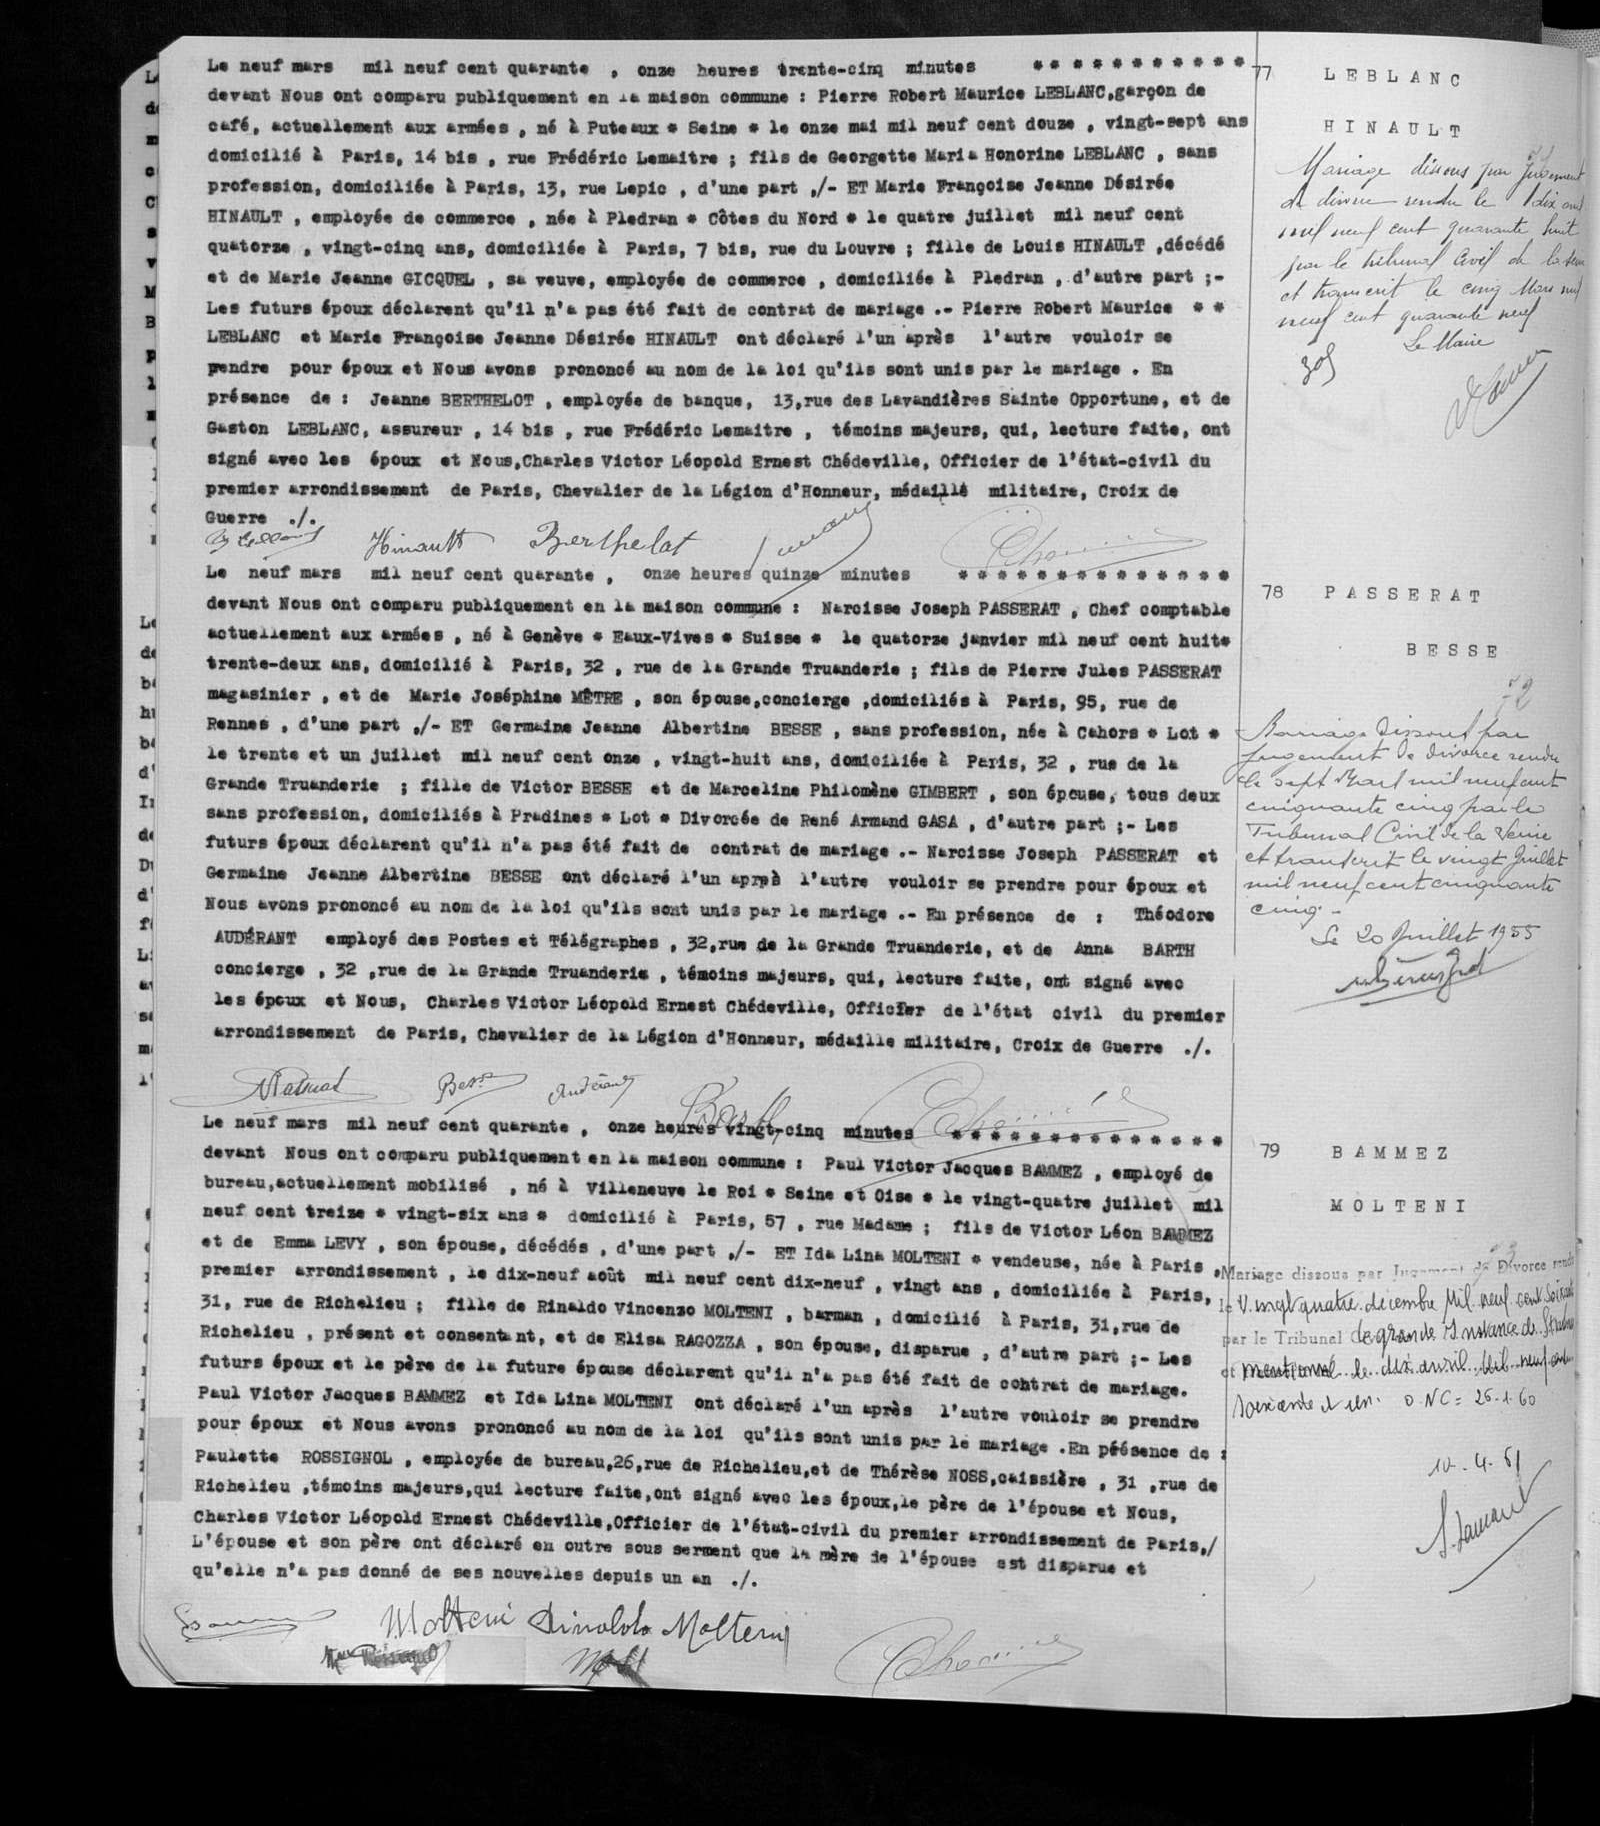Le neuf mars mil neuf cent quarante, onze heures trente-cinq minutes ****
devant Nous ont comparu publiquement en la maison commune: Pierre Robert Maurice LEBLANC, garçon de
café, actuellement aux armés, né à Puteux * Seine * le onze mai mil neuf cent douze, vingt-sept ans
domicilié à Paris, 14 bis, rue Frédéric Lemaitre; fils de Georgette Maria Honorine LEBLANC, sans
profession, domiciliée à Paris, 13, rue Lepic, d'une part ,/-ET Marie Françoise Jeanne Désirée
HINAULT, employée de commerce, née à Pledran * Côtes du Nord * le quatre juillet mil neuf cent
quatorze, vingt-cinq ans, domiciliée à Paris, 7 bis, rue du Louvre; fille de Louis HINAULT, décédé
et de Marie Jeanne GICQUEL, sa veuve, employée de commerce, domiciliée à Pledran, d'autre part;
Les futurs époux déclarent qu'il n'a pas été fait de contrat de mariage Pierre Robert Maurice **
LEBLANC et Marie Françoise Jeanne Désirée HINAULT ont déclaré l'un après l'autre vouloir se
prendre pour époux et Nous avons prononcé au nom de la loi qu'ils sont unis par le mariage. En
présence de: Jeanne BERTHELOT, employée de banque, 13, rue des Lavandières Sainte Opportune, et de
Gaston LEBLANC, assureur, 14 bis, rue Frédéric Lemaitre, témoins majeurs, qui, lecture faite, ont
signé avec les époux et Nous, Charles Victor Léopold Ernest Chédeville, Officier de l'état-civil du
premier arrondissement de Paris, Chevalier de la Légion d'Honneur, médaillé militaire, Croix de
Guerre ./.
Le neuf mars mil neuf cent quarante, onze heures quinze minutes ****
devant Nous ont comparu publiquement en la maison commune: Narcisse Joseph PASSERAT, Chef comptable
actuellement aux armées, né à Genève * Eaux-Vives * Suisse * le quatorze janvier mil neuf cent huit *
trente-deux ans, domicilié à Paris, 32, rue de la Grande Truanderie; fils de Pierre Jules PASSERAT
magasinier, et de Marie Joséphine METRE, son épouse, concierge, domiciliés à Paris, 95, rue de
Rennes, d'une part ,/-ET Germaine Jeanne Albertine BESSE, sans profession, née à Cahors * Lot *
le trente et un juillet mil neuf cent onze, vingt-huit ans, domiciliée à Paris, 32, rue de la
Grande-Truanderie; fille de Victor BESSE et de Marceline Philomène GIMBERT, son épouse, tous deux
sans profession, domiciliés à Pradines * Lot * Divorcéede rené Armand GASA, d'autre part ;-Les
futurs époux déclarent qu'il n'a pas été fait de contrat de mariage .-Narcisse Joseph PASSERAT et
Germaine Jeanne Albertine BESSE ont déclaré l'un après l'autre vouloir se prendre pour époux et
Nous avons prononcé au nom de la loi qu'ils sont unis par le mariage .-En présence de: Théodore
AUDERANT employé des Postes et Télégraphes, 32, rue de la Grande Truanderie, et de Anna BARTH
concierge, 32, rue de la Grande Truanderie, témoins majeurs, qui, lecture faite, ont signé avec
les époux et Nous, Charles Victor Léopold Ernest Chédeville, Officier de l'état civil du premier
arrondissement de Paris, Chevalier de la Légion d'Honneur, médaille militaire, Croix de Guerre ./.
Le neuf mars mil neuf cent quarante, onze heures vingt-cinq minutes ****
devant Nous ont comparu publiquement en la maison commune: Paul Victor Jacques BAMMEZ, employé de
bureau, actuellement mobilisé, né à Villeneuve le Roi * Seine et Oise * le vingt-quatre juillet mil
neuf cent treize * vingt-six ans * domicilié à Paris, 57, rue Madame; fils de Victor Léon BAMMEZ
et de Emma LEVY, son épouse, décédés, d'une part ,/-ET Ida Lina MOLTENI * vendeuse, née à Paris,
premier arrondissement, le dix-neuf août mil neuf cent dix-neuf, vingt ans, domiciliée à Paris,
31, rue de Richelieu; fille de Rinaldo Vincenzo MOLTENI, barman, domicilié à Paris, 31, rue de
Richelieu, présent et consentant, et de Elisa RAGOZZA, son épouse, disparue, d'autre part ;-Les
futurs époux et le père de la future épouse déclarent qu'il n'a pas été fait de contrat de mariage.
Paul Victor Jacques BAMMEZ et Ida Lina MOLTENI ont déclaré l'un après l'autre vouloir se prendre
pour époux et Nous avons prononcé au nom de la loi qu'ils sont unis par le mariage. En présence de:
Paulette ROSSIGNOL, employée de bureau, 26, rue de Richelieu, et de Thérèse NOSS, caissière, 31, rue de
Richelieu, témoins majeurs, qui lecture faite, ont signé avec les époux, le père de l'épouse et Nous,
Charles Victor Léopold Ernest Chédeville, Officier de l'état-civildi premier arrondissement de Paris ,/
L'épouse et son père ont déclaré en outre sous serment que la mère de l'épouse est disparue et
qu'elle n'a pas donné de ses nouvelles depuis un an ./.
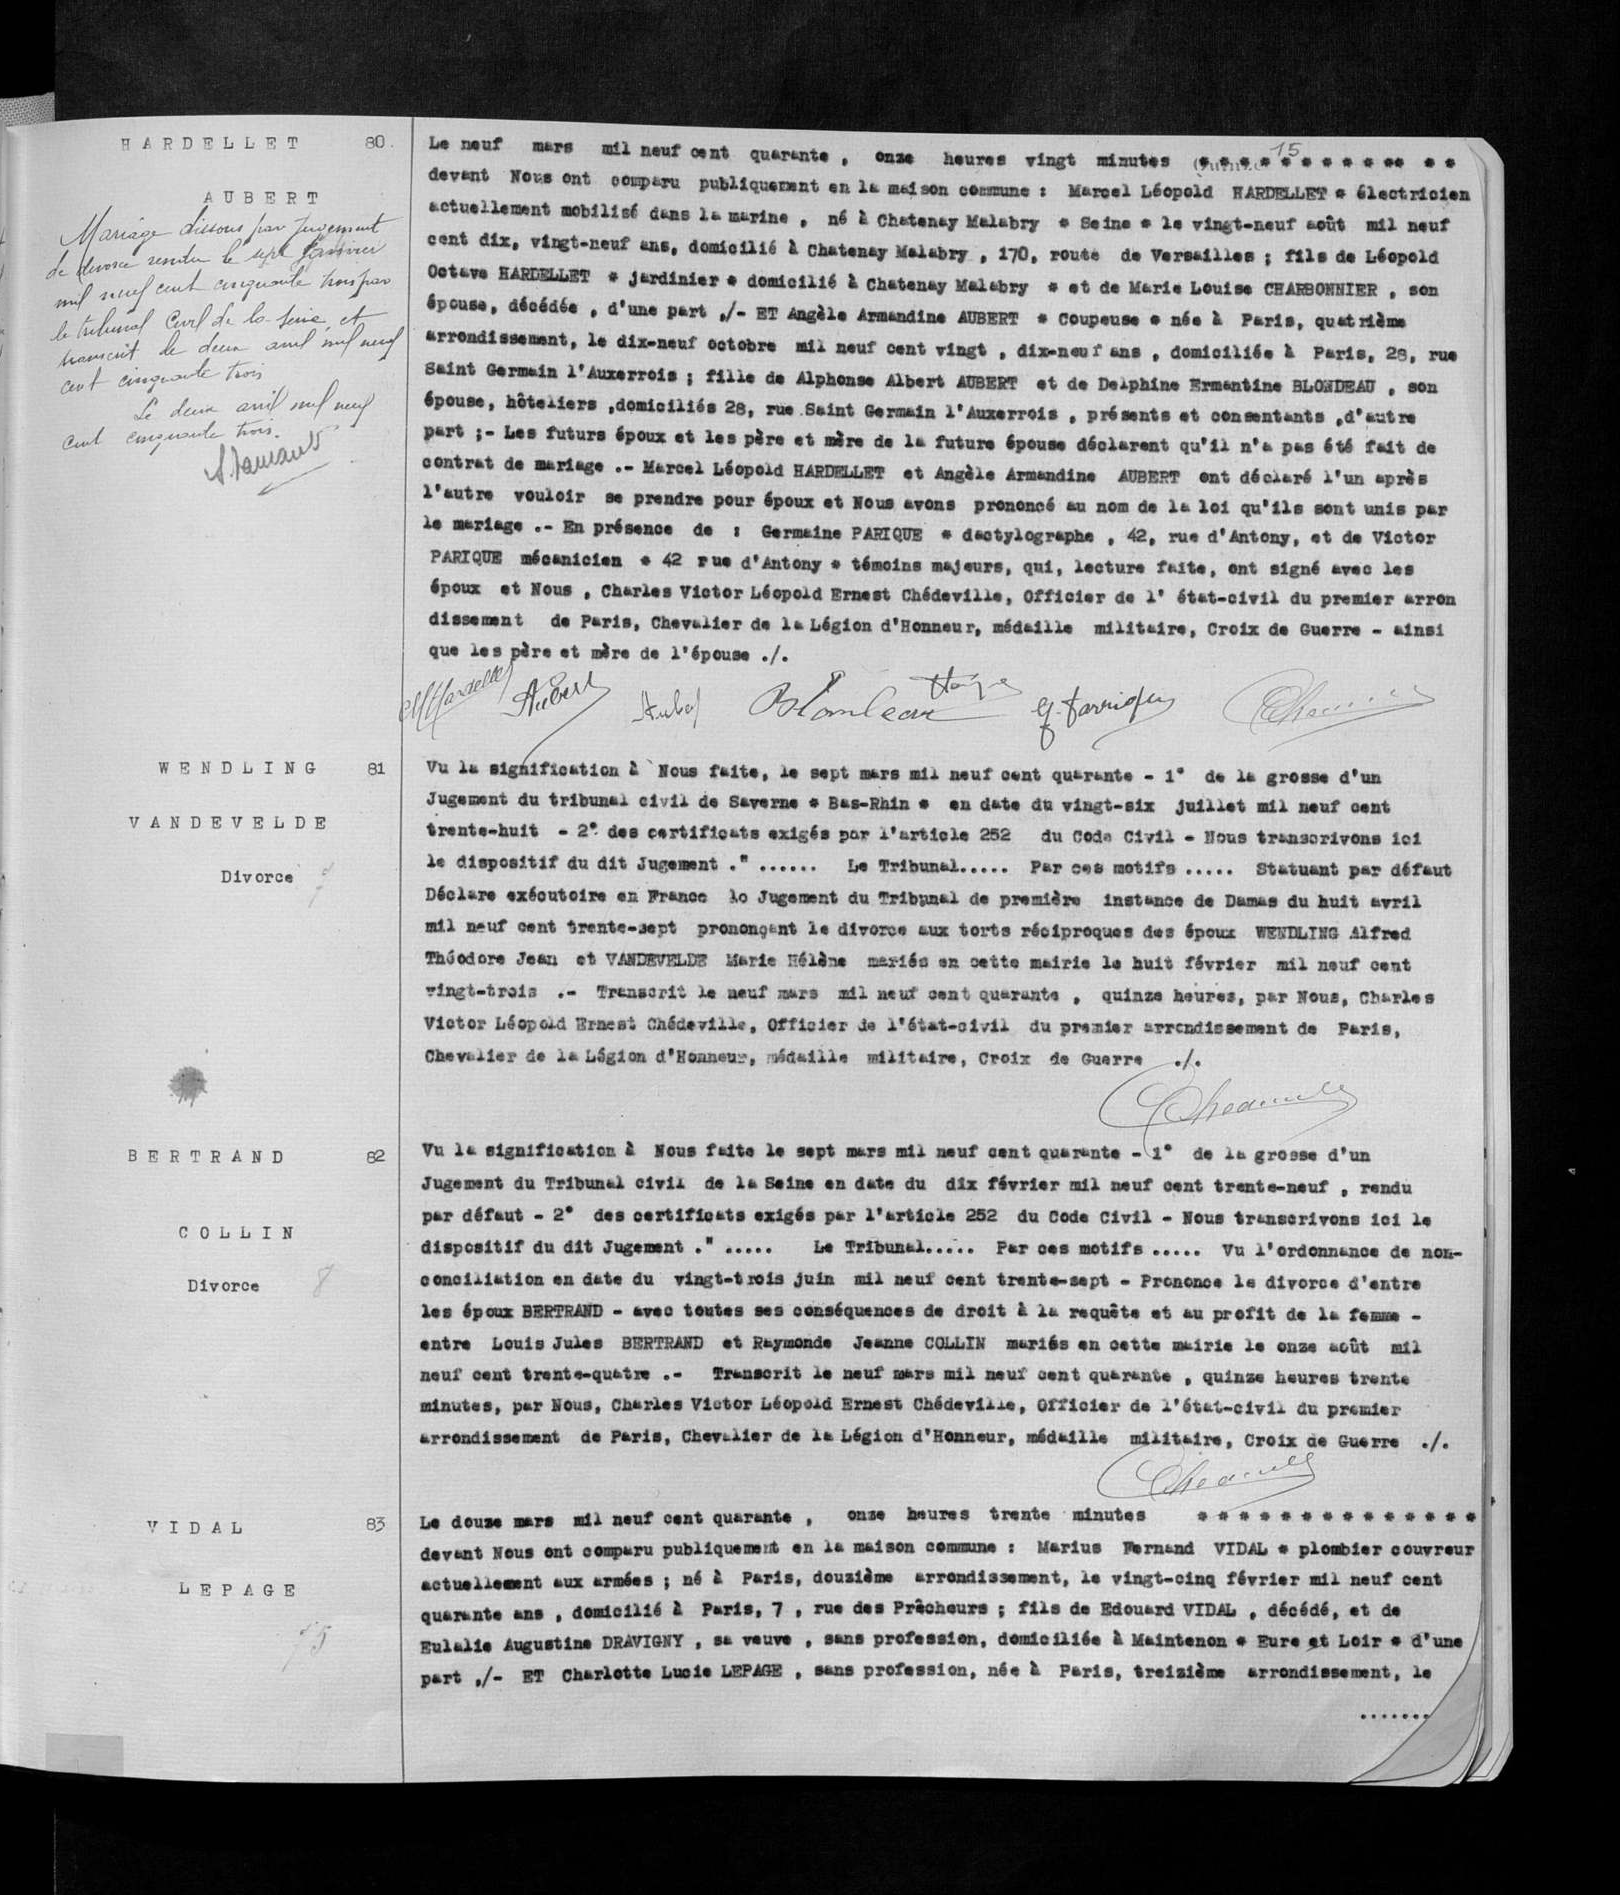Le neuf mars mil neuf cent quarante, onze heures vingt minutes ****
devant Nous ont comparu publiquement en la maison commune: Marcel Léopold HARDELLET * électricien
actuellement mobilisé dans la marine, né à Chatenay Malabry * Seine * le vingt-neuf août mil neuf
cent dix, vingt-neuf ans, domicilié à Chatenay Malabry, 170, route de Versailles; fils de Léopold
Octave HARDELLET * jardinier * domicilié à Chatenay Malabry * et de Marie Louise CHARBONNIER, son
épouse, décédée, d'une part ,/-ET Angèle Armandine AUBERT * Coupeuse * née à Paris, quatrième
arrondissement, le dix-neuf octobre mil neuf cent vingt, dix-neuf ans, domiciliée à Paris, 28, rue
Saint Germain l'Auxerrois; fille de Alphonse Albert AUBERT et de Delphine Ermantine BLONDEAU, son
épouse, hôteliers, domiciliés 28, rue Saint Germain l'Auxerrois, présents et consentants, d'autre
part ;-Les futurs époux et les père et mère de la future épouse déclarent qu'il n'a pas été fait de
contrat de mariage .-Marcel Léopold HARDELLET et Angèle Armandine AUBERT ont déclaré l'un après
l'autre vouloir se prendre pour époux et Nous avons prononcé au nom de la loi qu'ils sont unis par
le mariage .-En présence de: Germaine PARIQUE * dactylographe, 42, rue d'Antony, et de Victor
PARIQUE mécanicien * 42 rue d'Antony * témoins majeurs, qui, lecture faite, ont signé avec les
époux et Nous, Charles Victor Léopold Ernest Chédeville, Officier de l'état-civil du premier arron
dissement de Paris, Chevalier de la Légion d'Honneur, médaille militaire, Croix de Guerre-ainsi
que les père et mère de l'épouse ./.
Vu la signification à Nous faite, le sept mars mil neuf cent quarante-1° de la grosse d'un
Jugement du tribunal civil de Saverne * Bas-Rhin * en date du vingt-six juillet mil neuf cent
trente-huit-2° des certificats exigés par l'article 252 du Code Civil-Nous transcrivons ici
le dispositif du dit Jugement. '' ..... Le Tribunal..... Par ces motifs..... Statuant par défaut
Déclare exécutoire en France lo Jugement du Tribunal de première instance de Damas du huit avril
mil neuf cent trente-sept prononçant le divorce aux torts réciproques des époux WENDLING Alfred
Théodore Jean et VANDEVELDE Marie Hélène mariés en cette mairie le huit février mil neuf cent
vingt-trois .-Transcrit le neuf mars mil neuf cent quarante, quinze heures, par Nous, Charles
Victor Léopold Ernest Chédeville, Officier de l'état-civil du premier arrondissement de Paris,
Chevalier de la Légion d'Honneur, médaille militaire, Croix de Guerre ./.
Vu la signification à Nous faite le sept mars mil neuf cent quarante-1° de la grosse d'un
Jugement du Tribunal Civil de la Seine en date du dix février mil neuf cent trente-neuf, rendu
par défaut-2° des certificats exigés par l'article 252 du Code Civil-Nous transcrivons ici le
dispositif dudit Jugement. ''..... Le Tribunal..... Par ce motifs..... Vu l'ordonnance de non-
conciliation en date du vingt-trois juin mil neuf cent trente-sept-Prononce le divorce d'entre
les époux BERTRAND-avec toutes ses conséquences de droit à la requête et au profit de la femme-
entre Louis Jules BERTRAND et Raymonde Jeanne COLLIN mariés en cette mairie le onze août mil
neuf cent trente-quatre .-Transcrit le neuf mars mil neuf cent quarante, quinze heures trente
minutes, par Nous, Charles Victor Léopold Ernest Chédeville, Officier de l'état-civil du premier
arrondissement de Paris, Chevalier de la Légion d'Honneur, médaille militaire, Croix de Guerre ./.
Le douze mars mil neuf cent quarante, onze heures trente minutes ****
devant Nous ont comparu publiquement en la maison commune: Marius Fernand VIDAL * plombier couvreur
actuellement aux armées; né à Paris, douzième arrondissement, le vingt-cinq février mil neuf cent
quarante ans, domicilié à Paris, 7, rue des Prêcheurs; fils de Edouard VIDAL, décédé, et de
Eulalie Augustine DRAVIGNY, sa veuve, sans profession, domiciliée à Maintenon * Eure et Loir * d'une
part ,/-ET Charlotte Lucie LEPAGE, sans profession, née à Paris, treizième arrondissement, le